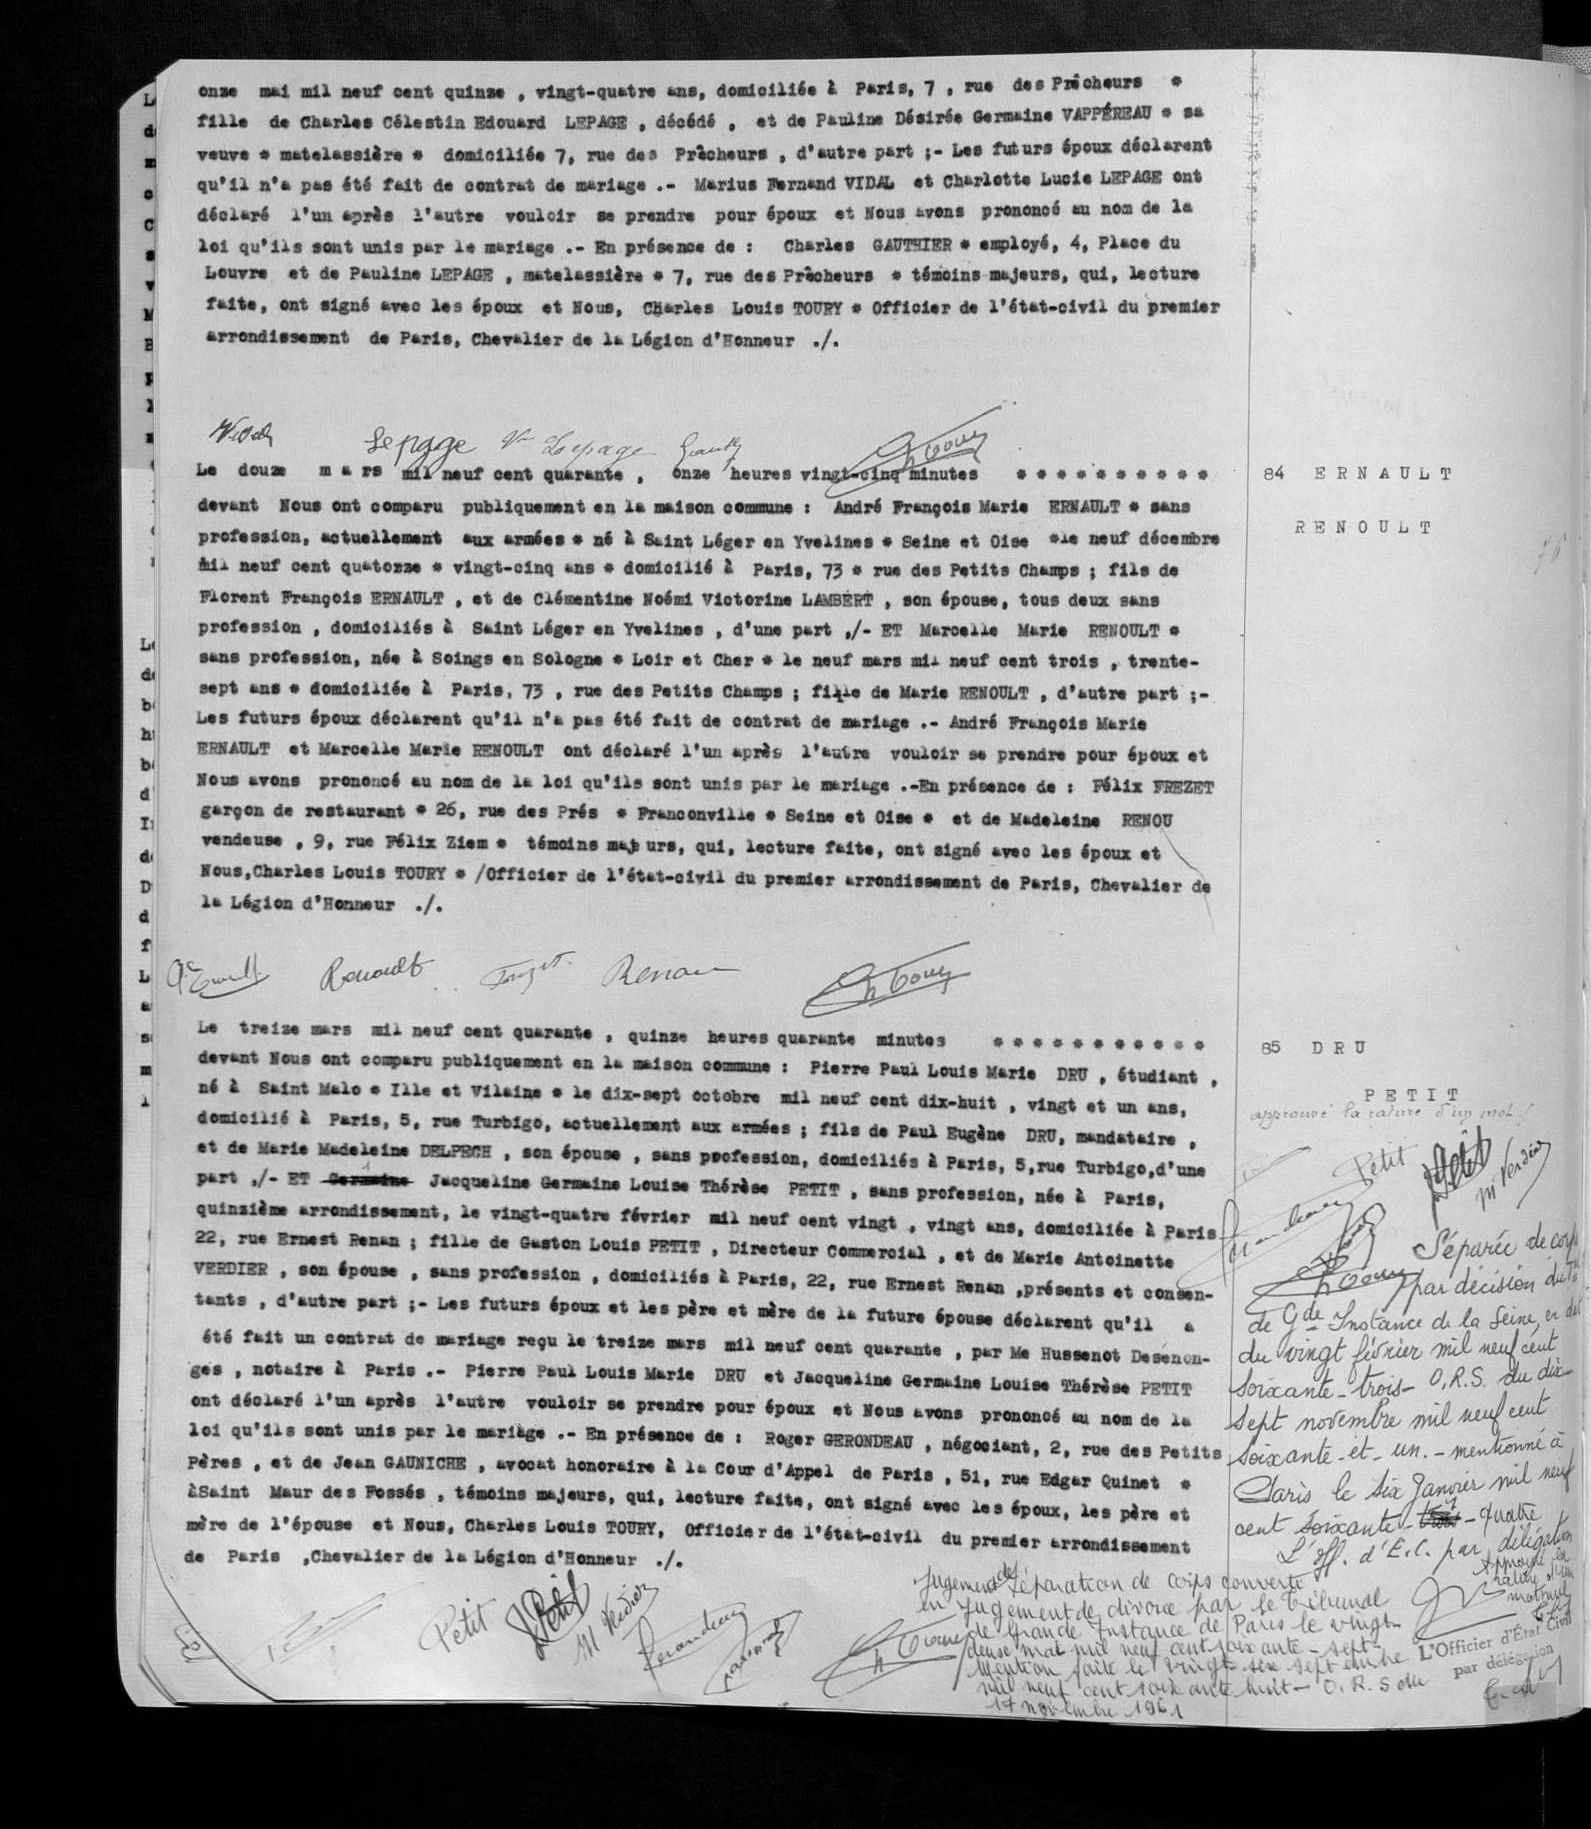onze mai mil neuf cent quinze, vingt-quatre ans, domiciliée à Paris, 7, rue des Prêcheurs *
fille de Charles Célestin Edouard LEPAGE, décédé, t de Pauline Désirée Germain VAPPEREAU * sa
veuve * matelassière * domiciliée 7, rue des Prêcheurs, d'autre part ;-Les futurs époux déclarent
qu'il n'a pas été fait de contrat de mariage .-Marius Fernand VIDAL et Charlotte Lucie LEPAGE ont
décalé l'un après l'autre vouloir se prendre pour époux et Nous avons prononcé au nom de la
loi qu'ils sont unis par le mariage .-En présence de: Charles GAUTHIER * employé, 4, Place du
Louvre et de Pauline LEPAGE, matelassière * 7, rue des Prêcheurs * témoins majeurs, qui, lecture
faite, ont signé avec les époux et Nous, Charles Louis TOURY * Officier de l'état-civil du premier
arrondissement de Paris, Chevalier de la Légion d'Honneur ./.
Le douze mars mil neuf cent quarante, onze heures vingt-cinq minutes ****
devant Nous ont comparu publiquement en la maison commune: André François Marie ERNAULT * sans
profession, actuellement aux armées * né à Saint Léger en Yvelines * Seine et Oise * le neuf décembre
mil neuf cent quatorze * vingt-cinq ans * domicilié à Paris, 73 * rue des Petits Champs; fils de
Florent François ERNAULT, et de Clémentine Noémi Victorine LAMBERT, son épouse, tous deux sans
profession, domiciliés à Saint Léger en Yvelines, d'une part ,/-ET Marcelle Marie RENOULT *
sans profession, née à Soings en Sologne * Loir et Cher * le neuf mars mil neuf cent trois, trente-
sept ans * domiciliée à Paris, 73, rue des Petits Champs; fille de Marie RENOULD, d'autre part;
Les futur époux déclarent qu'il n'a pas été fait de contrat de mariage .-André François Marie
ERNAULT et Marcelle Marie RENOULT ont déclaré l'un après l'autre vouloir se prendre pou époux et
Nous avons prononcé au nom de la loi qu'ils sont unis par le mariage .-En présence de: Félix FREZET
garçon de restaurant * 26, rue des Prés * Franconville * Seine et Oise * et de Madeleine RENOU
vendeuse, 9, rue Félix Ziem * témoins maje urs, qui, lecture faite, ont signé avec les époux et
Nous, Charles Louis TOURY */ Officier de l'état-civil du premier arrondissement de Paris, Chevalier de
la Légion d'Honneur ./.
Le treize mars mil neuf cent quarante, quinze heures quarante minutes ****
devant Nous ont comparu publiquement en la maison commune: Pierre Paul Louis Marie DRU, étudiant,
né à Saint Malo * Ille et Vilaine * le dix-sept octobre mil neuf cent dix-huit, vingt et un ans,
domicilié à Paris, 5, rue Turbigo, actuellement aux armées; fils de Paul Eugène DRU, mandataire,
et de Marie Madeleine DELPECH, son épouse, sans profession, domiciliés à Paris, 5, rue Turbigo, d'une
part ,/-ET Germaine Jacqueline Germaine Louise Thérèse PETIT, sans profession, née à Paris,
quinzième arrondissement, le vingt-quatre février mil neuf cent vingt, vingt ans, domiciliée à Paris
22, rue Ernest Renan; fille de Guston Louis PETIT, Directeur Commercial, et de Marie Antoinette
VERDIER, son épouse, sans profession, domiciliés à Paris, 22, rue Ernest Renan, présents et consen-
tant, d'autre part ;-Les futurs époux et les père et mère de la future épouse déclarent qu'il a
été fait un contrat de mariage reçu le treize mars mil neuf cent quarante, par Me Hussenot Desenon-
ges, notaire à Paris .-Pierre Paul Louis Marie DRU et Jacqueline Germaine Louise Thérèse PETIT
ont déclaré l'un après l'autre vouloir se prendre pour époux et Nous avons prononcé au nom de la
loi qu'ils sont unis par le mariage .-En présence de: Roger GERONDEAU, négociant, 2, rue des Petits
Pères, et de Jean GAUNICHE, avocat honoraire à la Cour d'Appel de Paris, 51, rue Edgar Quinet *
à Saint Maure des Fossés, témoins majeurs, qui, lecture faite, ont signé avec les époux, les père et
mère de l'épouse et Nous, Charles Louis TOURY, Officier de l'état-civil du premier arrondissement
de Paris, Chevalier de la Légion d'Honneur ./.
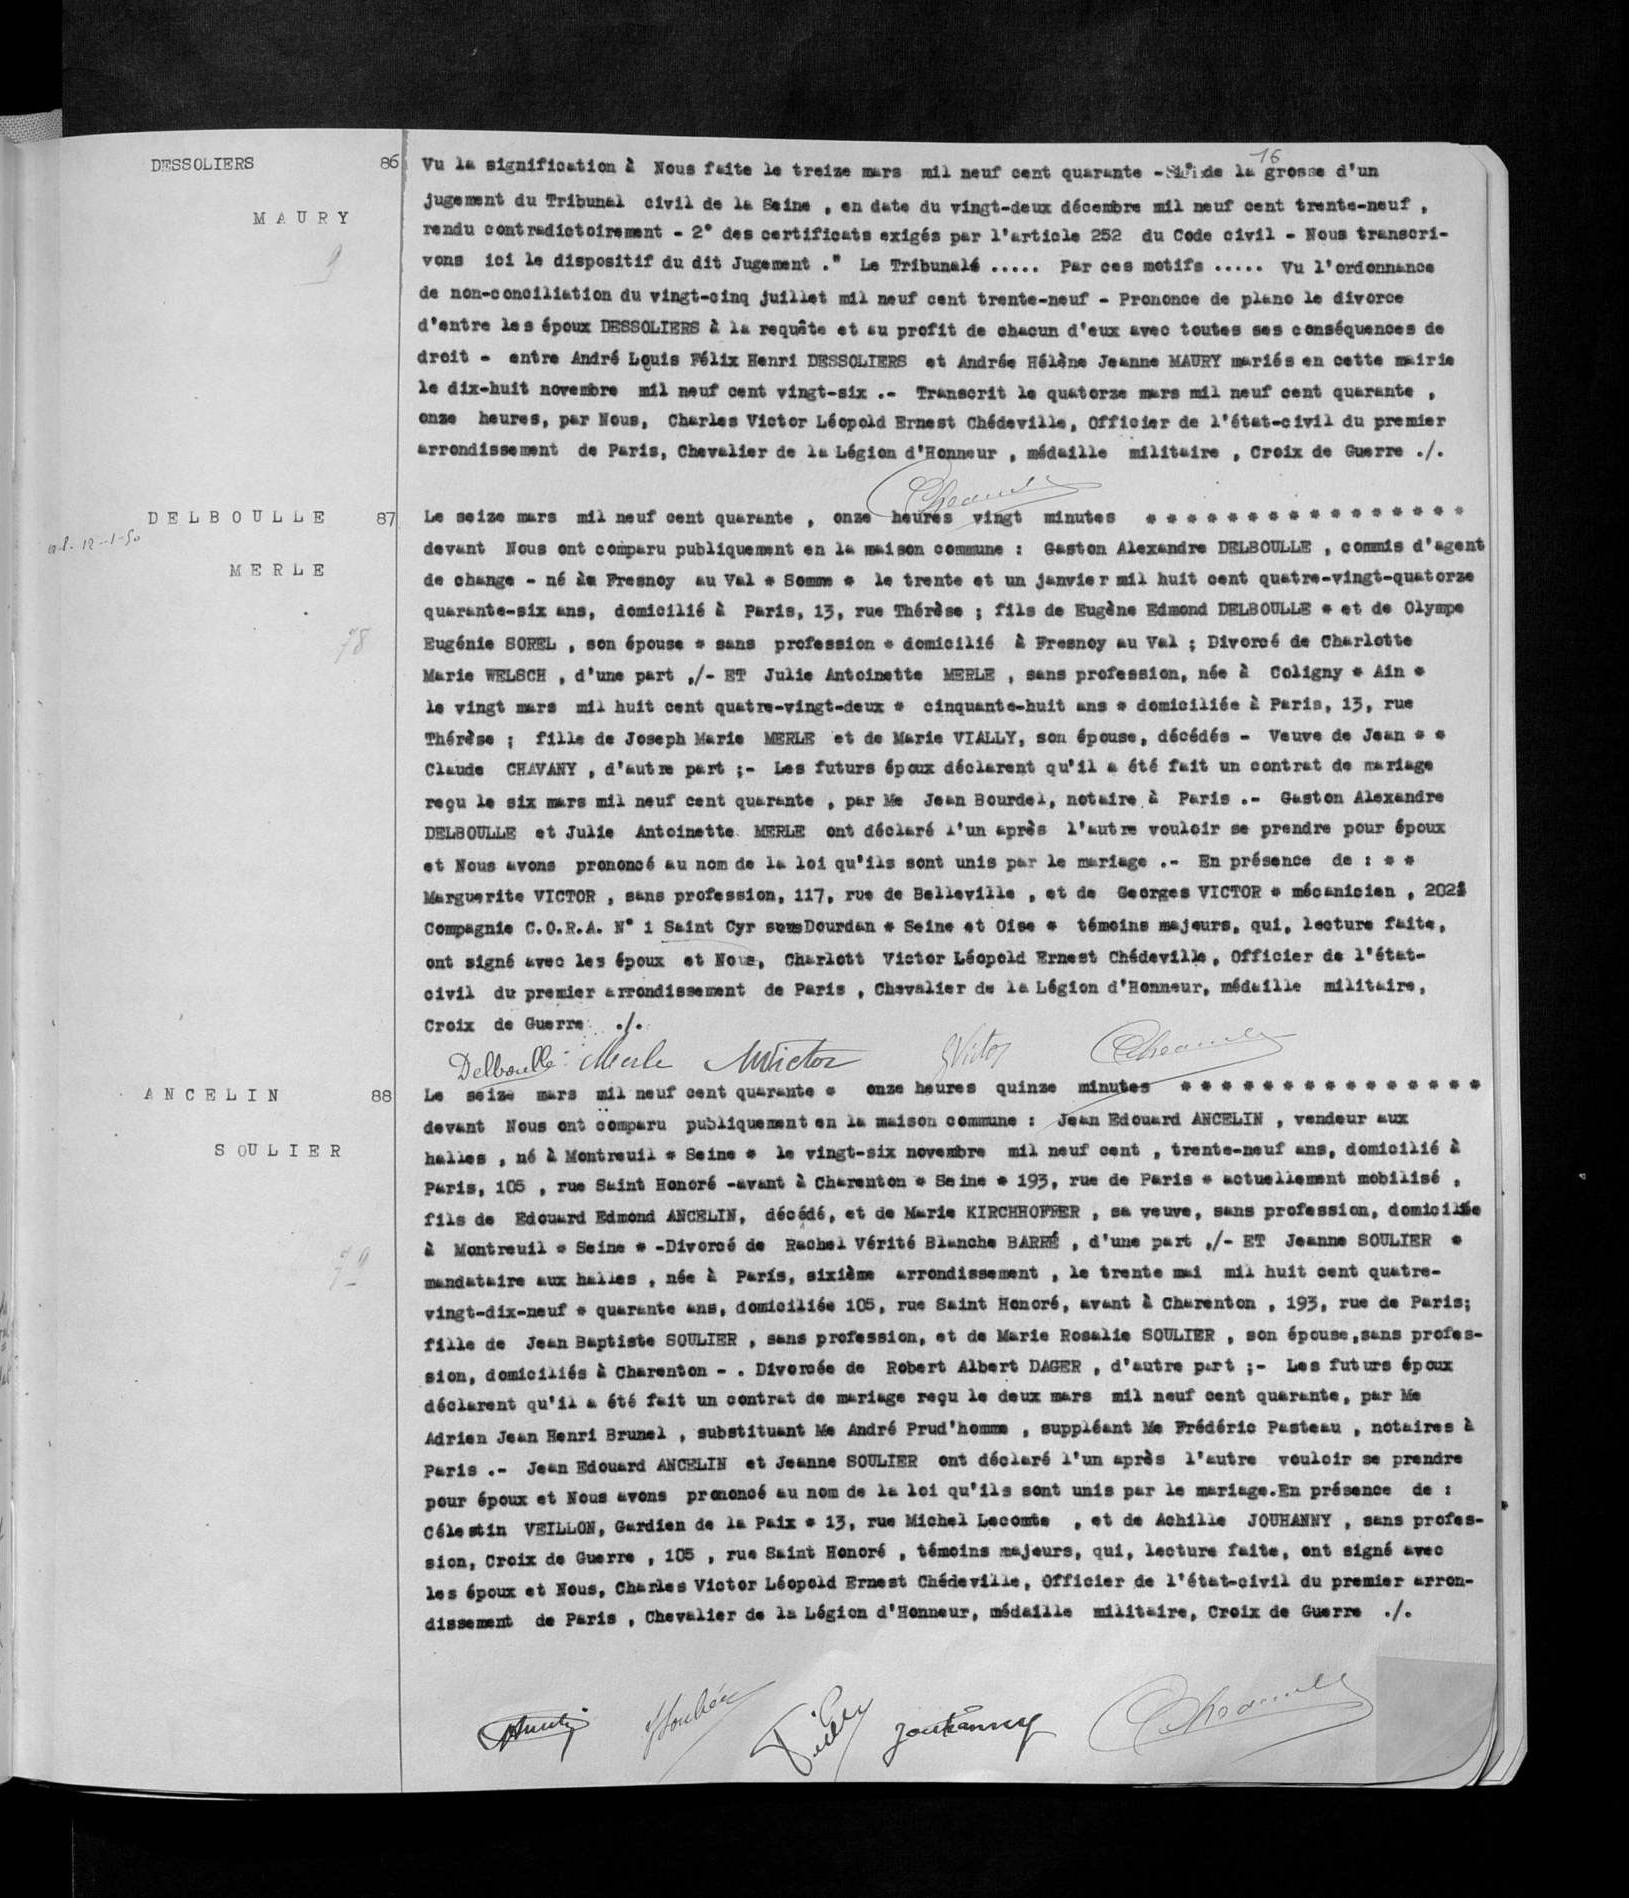Vu la signification à Nous faite le treize mars mil neuf cent quarante-Sleide de la grosse d'un
jugement du Tribunal civil de la Seine, en date du vingt-deux décembre mil neuf cent trente-neuf,
rendu contracdictoirement-2° des certificats exigés par l'article 252 du Code civil-Nous transcri-
vons ici le dispositif du dit Jugement. ''Le Tribunalé..... Par ces motifs.... Vu l'ordonnance
de non-conciliation du vingt-cinq juillet mil neuf cent trente-neuf-Prononce de planc le divorce
d'entre les époux DESSOLIERS à la requête et au profit de chacun d'eux avec toutes ses conséquences de
droit-entre André Louis Félix Henri DESSOLIERS et Andrée Hélène Jeanne MAURY mariés en cette mairie
le dix-huit novembre mil neuf cent vingt-six .-Transcrit le quatorze mars mil neuf cent quarante,
onze heures, par Nous, Charles Victor Léopold Ernest Chédeville, Officier de l'état-civil du premier
arrondissement de Paris, Chevalier de la Légion d'Honneur, médaille militaire, Croix de Guerre ./.
Le seize mars mil neuf cent quarante, onze heures vingt minutes ****
devant Nous ont comparu publiquement en la maison commune: Gaston Alexandre DELBOULLE, commis
d'agent de change-né à * Fresnoy au Val * Somme * le trente et un janvier mil huit cent quatre-vingt-quatorze
quarante-six ans, domicilié à Paris, 13, rue Thérèse; fils de Eugène Edmond DELBOULE * et de Olympe
Eugènie SOREL, son épouse * sans profession * domicilié à Fresnoy au Val; Divorcé de Charlotte
Marie WELSCH, d'une part ,/-ET Julie Antoinette MERLE, sans profession, née à Coligny * Ain *
le vingt mars mil huit cent quatre-vingt-deux * cinquante-huit ans * domiciliée à Paris, 13, rue
Thérèse; fille de Joseph Marie MERLE et de Marie VIALLY, son épouse, décédés-Veuve de Jean **
Claude CHAVANY, d'autre part ;-Les futurs époux déclarent qu'il a été fait un contrat de mariage
reçu le six mars mil neuf cent quarante, par Me Jean Bourdel, notaire à Paris .-Gaston Alexandre
DELBOULE et Julie Antoinette MERLE ont déclaré l'un après l'autre vouloir se prendre pour époux
et Nous avons prononcé au nom de la loi qu'ils sont unis par le mariage .-En présence de: **
Marguerite VICTOR, sans profession, 117, rue de Belleville, et de Georges VICTOR * mécanicien, 202
Compagnie C. O. R. A. N ° 1 Saint Cyr sous Dourdan * Seine et Oise * témoins majeurs, qui, lecture faite,
ont signé avec les époux et Nous, Charlott Victor Léopold Ernest Chédeville, Officier de l'état-
civil du premier arrondissement de Paris, Chevalier de la Légion d'Honneur, médaille militaire,
Croix de Guerre ./.
Le seize mars mil neuf cent quarante * onze heures quinze minutes ****
devant Nous ont comparu publiquement en la maison commune: Jean Edouard ANCELIN, vendeur aux
halles, né à Montreuil * Seine * le vingt-six novembre mil neuf cent, trente-neuf ans, domicilié à
Paris, 105, rue Saint Honoré-avant à Charenton * Seine * 193, rue de Paris * actuellement mobilisé,
fils de Edouard Edmond ANCELIN, décédé, et de Marie KIRCHHOFEER, sa veuve, sans profession, domiciliée
à Montreuil * Seine *-Divorcé de Rachel Vérité Blanche BARRE, d'une part ,/-ET Jeanne SOULIER *
mandataire aux halles, née à Paris, sixième arrondissement, le trente mai mil huit cent quatre
vingt-dix-neuf * quarante ans, domiciliée 105, rue Saint Honoré, avant à Charenton, 193, rue de Paris;
fille de Jean Baptise SOULIER, sans profession, et de Marie Rosalie SOULIER, son épouse, sans profes-
sion, domiciliés à Charenton -. Divorcée de Robert Albert DAGER, d'autre part ;-Les futurs époux
déclarent qu'il a été fait un contrat de mariage reçu le deux mars mil neuf cent quarante, par Me
Adrien Jean Henri Brunel, substituant Me André Prud'homme, suppléant Me Frédéric Pasteau, notaires à
Paris .-Jean Edouard ANCELIN et Jeanne SOULIER ont déclaré l'un après l'autre vouloir se prendre
pour époux et Nous avons prononcé au nom de la loi qu'ils sont unis par le mariage. En présence de:
Célestin VEILLON, Gardien de la Paix * 13, rue Michel Lecomte, et de Achille JOUHANNY, sans profes-
sion, Croix de Guerre, 105, rue Saint Honoré, témoins majeurs, qui, lecture faite, ont signé avec
les époux et Nous, Charles Victor Léopold Ernest Chédeville, Officier de l'état-civil du premier arron-
dissement de Paris, Chevalier de la Légion d'Honneur, médaille militaire, Croix de Guerre ./.